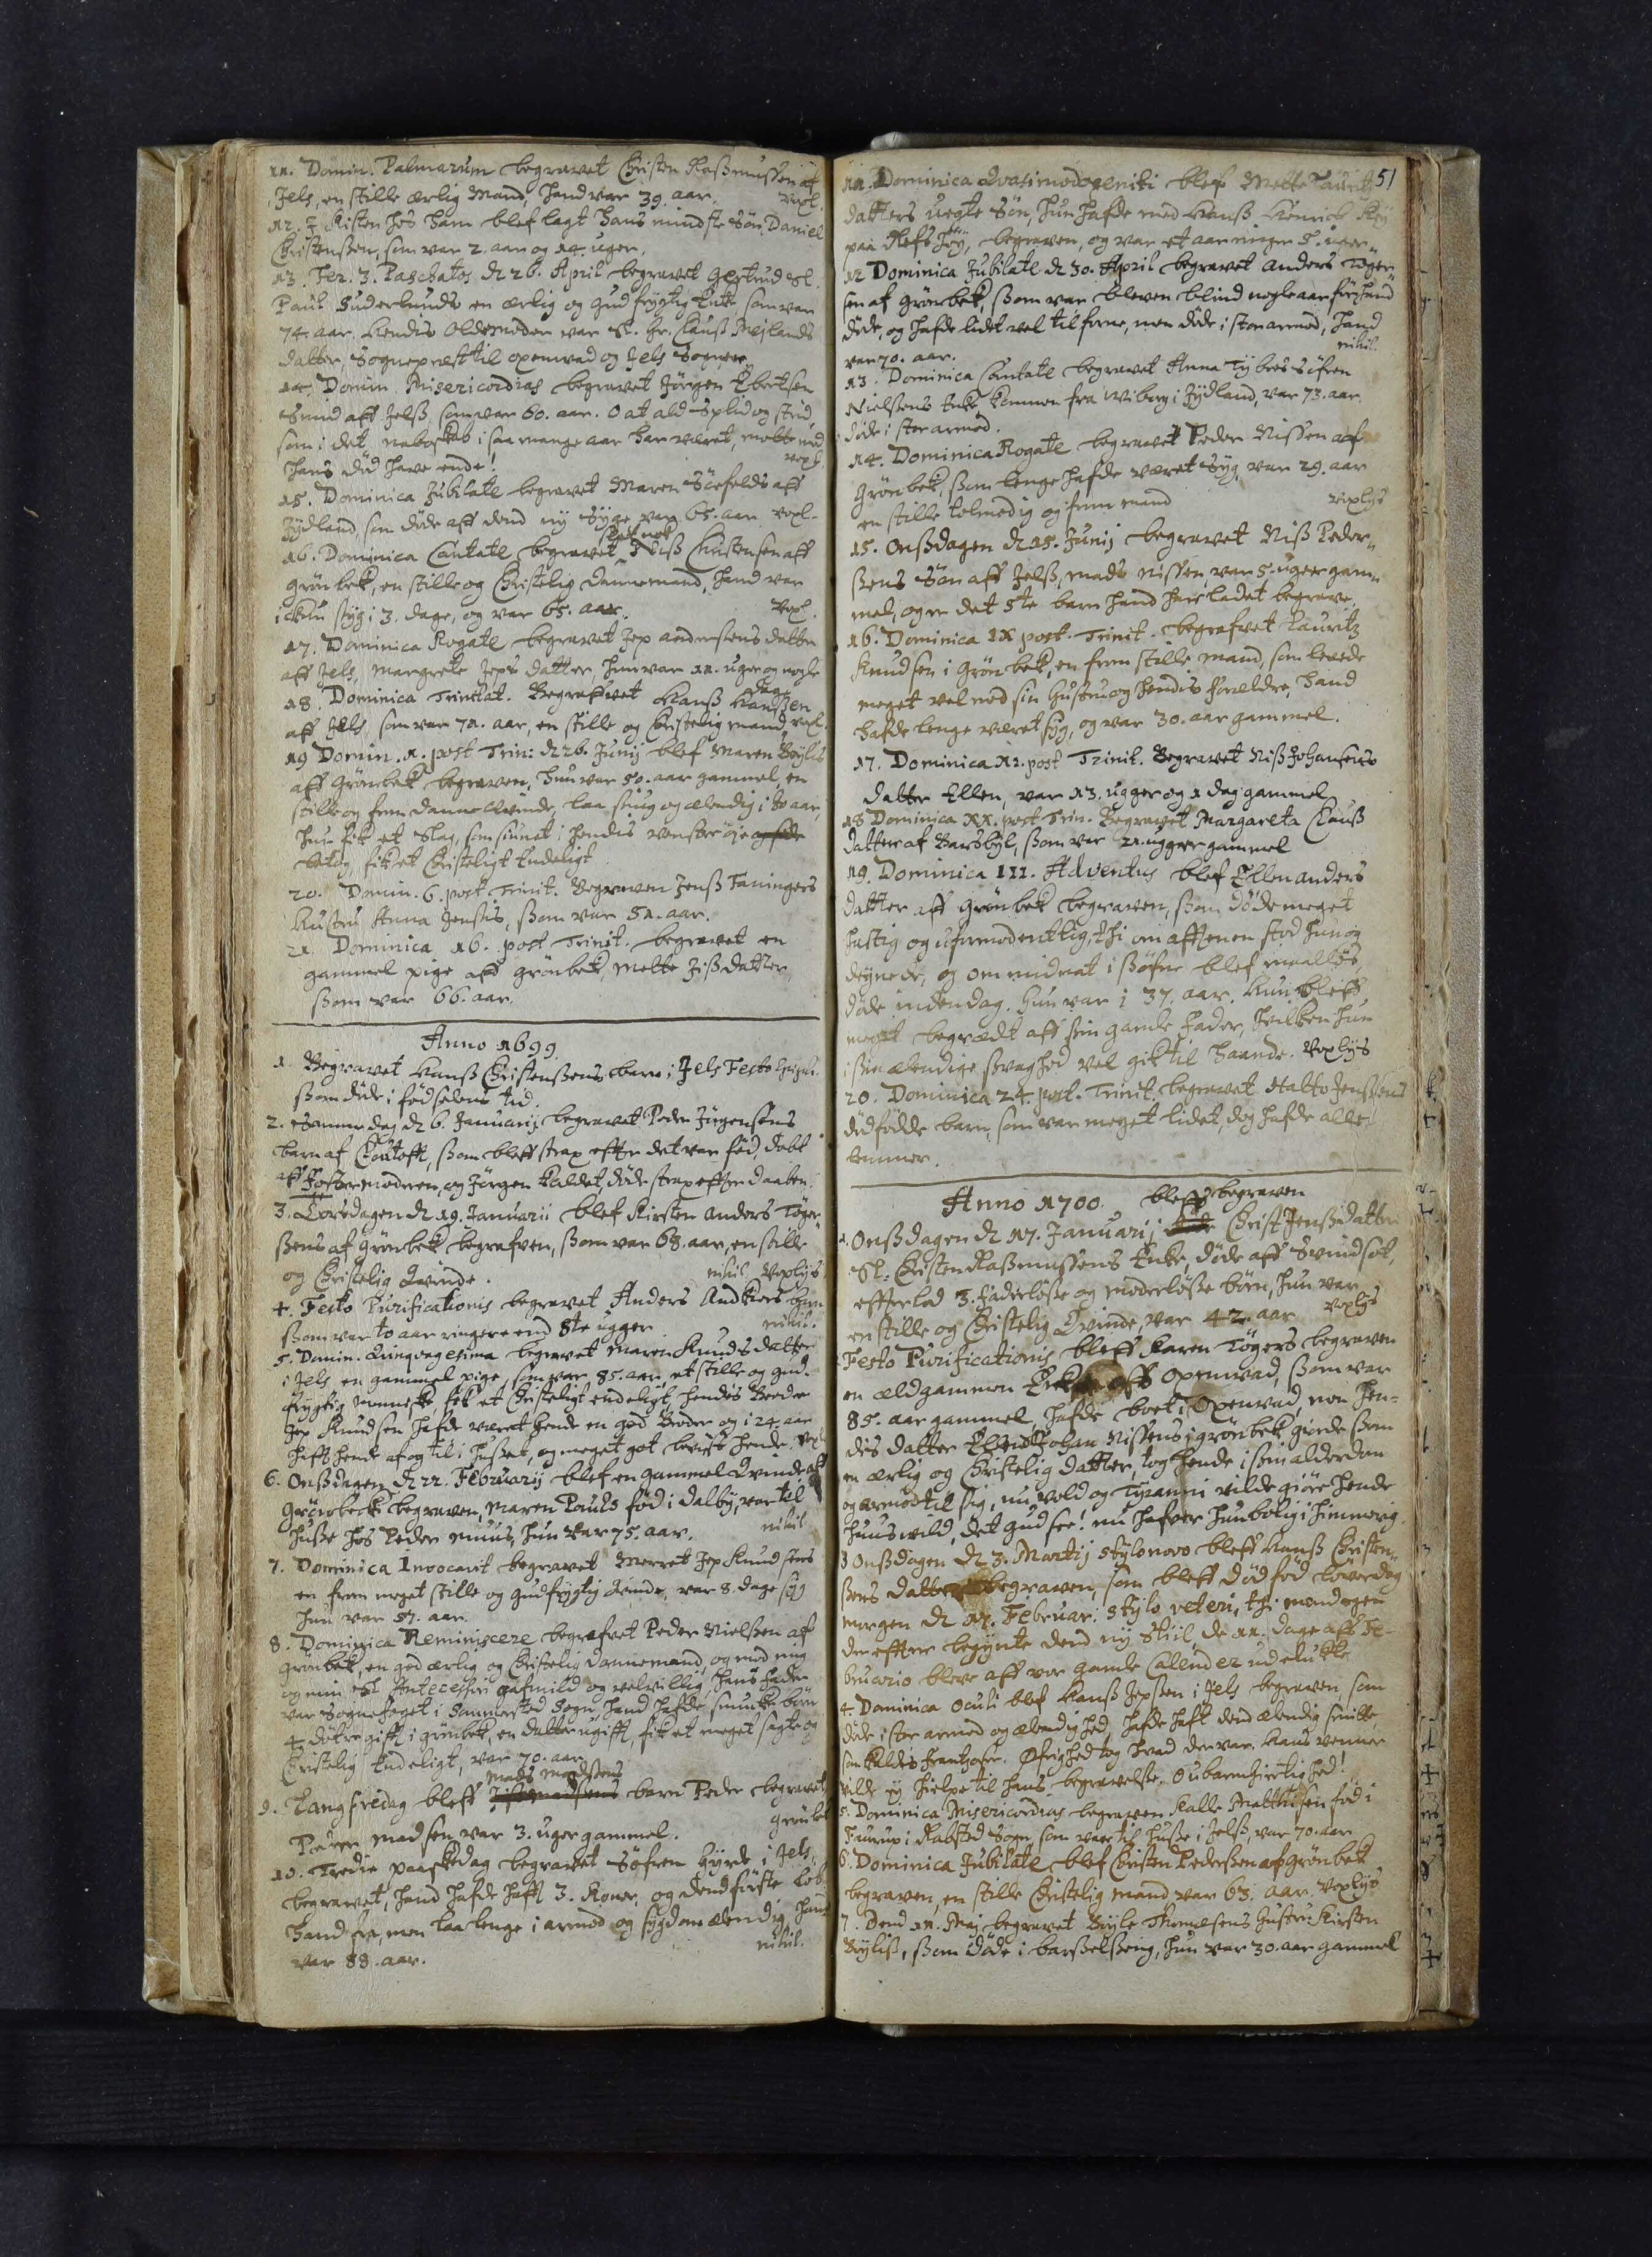11. Domin. Palmarum begravet Christen Rassmussen af
Jels, en stille ærlig Mand, hand var 39 aar Voxl.
12. I kisten hos ham blef lagt hans mindste Søn Daniel
Christensen, som var 2. aar og 14. uger.
13. Fer. 3. Paschatos d 26. April begravet Gertrud Sl.
Paul Suderlunds en ærlig og gud frygtig Enke, som var
74. aar. Hendis Oldemoder var Sl. Hr. Claus Mejlands
datter, Sognepræst til Oksenvad og Jels Sogne.
14. Domin. Misericordias begravet Jørgen Ebertsen
Smid aff Jelss som var 60. aar. O at alders splid og strid,
som i det naboskab i saa mange aar har veret, motte med
hans død have ende
voxl.
15. Dominica Jubilate begravet Maren Søefelds aff
Jydland, som døde aff dend ny Syge var 65. aar voxl.
sl##t nok##
16. Dominica Cantate begravet Niss Christensen aff
Grønbek, en stille og Christelig Dannemand, hand var
ickun syg i 3. aar, og var 65. aar. Voxl.
17. Dominica Rogate begravet Jep Andersens datter
aff Jels, Margrete Jeps datter, hun var 11. uger og nogle
dage
18. Dominica Trinitat. Begrafdfvet Hanss Hanssen
aff Jels, som var 71. aar, en stille og Christelig Mand. voxl.
19. Domin. 1. post Trin: d 26. Junij blef Maren Boylis
aff Grønbek begraven, hun var 50. aar gammel, en
stille og from Danne Qvinde, laa Siug og ælendig i to aar,
hun fik et Slag, som siunet i hendis venster øje og
betog, fik et Christeligt Endeligt
20. Domin. 6. post. Trinit. Begraven Jenss Faningers
Hustru Anna Jensis, ssom var 51. aar.
21. Dominica 16. post Trinit. begravet en
gammel pige aff Grønbek, Mette Jissdatter
ssom var 66. aar.
Anno 1699.
1. Begravet Hanss Christenssens barn i Jels Festo Epiph.
ssom døde i fødselens tid.
2. Samme dag d 6 Januarij begravet Peder Jørgensens
barn aff Cloutofft, som bleff strax efter det var fød, døbt
aff Fostermoderen, og Jørgen kaldet, døde strax efter daaben
3. Torsdagen d 19. Januarii blef Kirsten Anders Tøger
ssens aff Grønbek begrafven, ssom var 68. aar, en stille
og Christelig Qvinde. nihil voxlys.
+ 4. Festo Purificationis begravet Anders Andkiers barn,
ssom var to aar ringere end 8te ugger. nihil.
5. Domin. Qvinquagesima begravet Maren Knudsdatter
i Jels en gammel pige, som var 85. aar, et stille og gud¬
frygtig menneske, fik et Christeligt endeligt, hendis Broder
Jep Knudsen hafde veret Hende en god Broder og i 24. aar
hafft hende af og til i Huset, og meget got bevi##st hende. voxl
6. Onsdagen d 22. Februarij blef en gammel Qvinde aff
Grønbeck begraven, Maren Pouls fød i Dalby, var til
huse hos Peder Muus, hun var 75. aar. nihil
7. Dominica Invocavit begravet Merret Jep Knudsens
en from meget stille og gudfrygtig Qvinde, var 8 dage syg
hun var 57. aar.
8. Dominica Reminiscere begrafvet Peder Nielssen af
Grønbek, en god ærlig og Christelig Dannemand, og mod mig
og min Sl Antece## gafmild og velvillig, hans fader
var Sognefoget i Sommersted Sogn hand hafde smucke børn
4. døtre gifft i Grønbek, en datter ugift, fik et meget sagte og
Christelig Endeligt, var 70. aar
Mads Madsens
9. Langfredag bleff Jis Madssens barn Peder begravet
Peder Madsen var 3. uger gammel. Grønbek
10. Tredji paaskedag begravet Søfren Hyrde i Jels
begravet, hand hafde hafft 3. koner, og dend første løb
hand fra, men laa lenge i armod og sygdom ælendig hand
var 88. aar. nohil.
51
11. Dominica Qvasimodogeniti blef Mette Lauritz
datters uegte Søn, hun hafde med Hanss Henrich Key
paa Refs Høy, begraven, og var et aar ringer 5. uger.
12. Dominica Jubilate d 30. April begravet Anders Tøger¬
sen af Grønbek, ssom var bleven blind nogle aar før hand
døde, og hafde lidet vel tilforne, men døde i stor armod, hand
var 70. aar. nihil.
13. Dominica Cantate begravet Anna Tyboes Søfren
Nielssens Enke kommen fra Viborg i Jydland, var 73. aar.
døde i stor armod.
14. Dominica Rogate begravet Peder Nissen af
Grønbek, ssom lenge hafde været Syg, var 29. aar
en stille tolmodig og from mand. Voxlys
15. Onssdagen d 15. Junij begravet Niss Peder¬
sens Søn aff Jelss, Mads Nissen, var 5. uger gam¬
mel, og er det 5te barn hand har ladet begrave.
16. Dominica IX post Trinit. begrafvet Lauritz
Knudsen i Grønbek, en from stille mand, som levede
meget vel med sin Hustru og hendis foreldre, hand
hafde lenge veret syg, og var 30. aar gammel.
17. Dominica 12. post Trinit. Begravet Niss Johansens
datter Ellen, var 13. ugger og 1 dag gammel
18. Dominica XX. Post Trin. Begravet Margareta Clauss
datter aff Barsbyl, ssom var 21. ugger gammel
19. Dominica III. Adventus blef Ellen Anders
datter aff Grønbek begraven, ssom døde meget
hastig og uformodentlig, thi om aftenen stod hun og
deynede##, og om midnat i søfne blef maaløs
døde inden dag. Hun var i 37. aar. Hun bleff 
meget begrædt aff sin gamle fader, hvilken hun
i ssin ælendige svaghed vel gik til haande. Voxlys
20. Dominica 24. post. Trinit begravet Hatto Jenssens
dødfødde barn, som var meget lidet, dog hafde alle
lemmer.
Anno 1700 bleff begraven
1. Onsdagen 17. Januarij Christ Jenssdatter
Sl. Christen Rassmussens Enke, døde aff Svindsot,
efsterlod 3. faderløsse og moderløsse børn, hun var
en stille og Christelig Qvinde, var 42. aar. Voxlys
Festo Purificationis bleff Karen Tøgers begraven
en ældgammen Enke aff Oxenvad, ssom var
85. aar gammel, hafde boet i Oxenvad, men hen¬
dis datter Ellen Johan Nissens i Grønbek giorde ssom
en ærlig og Christelig Datter, tog hende i ssin alderdom
og armod til ssig, nu vold og tyranni vilde giøre hende
huusvild, det Gud ser! Nu hafver hun bolig i himmerig.
3. Onssdagen d 3. Martij stylonovo bleff Hanss Christen¬
sens Datter ## begraven, som bleff dødfød løverdag
morgen d 17. Februar: stylo veteri, thi mandagen
dereffter begynte dend ny stiil, de 11. Dage aff Fe¬
bruario blev aff vor gamle Calender udelukte
4. Dominica Oculi blef Hanss Jepssen i Jels begraven, som
døde i stor armod og ælendighed, hafde haft dend ælendig smitte
som kaldis frantzoser. Øfrighed tog hvad der var. Hans venner
ville ey hielpe til hans begravelse. O ubarmhjertighed!
5. Dominica Misericordias begraven Kalle Matthisen fød i
Faurup i Rabsted Sogn, som var til husse i Jelss, var 70. aar
6. Dominica Jubilate blef Christen Pederssen af Grønbek
begraven, en stille Christelig mand, var 63. aar. Voxlys
7. Dend 11. May begravet Boyle Thomæsens Hustru Kirsten
Boyliss, ssom døde i barsselsseng, hun var 30. aar gammel